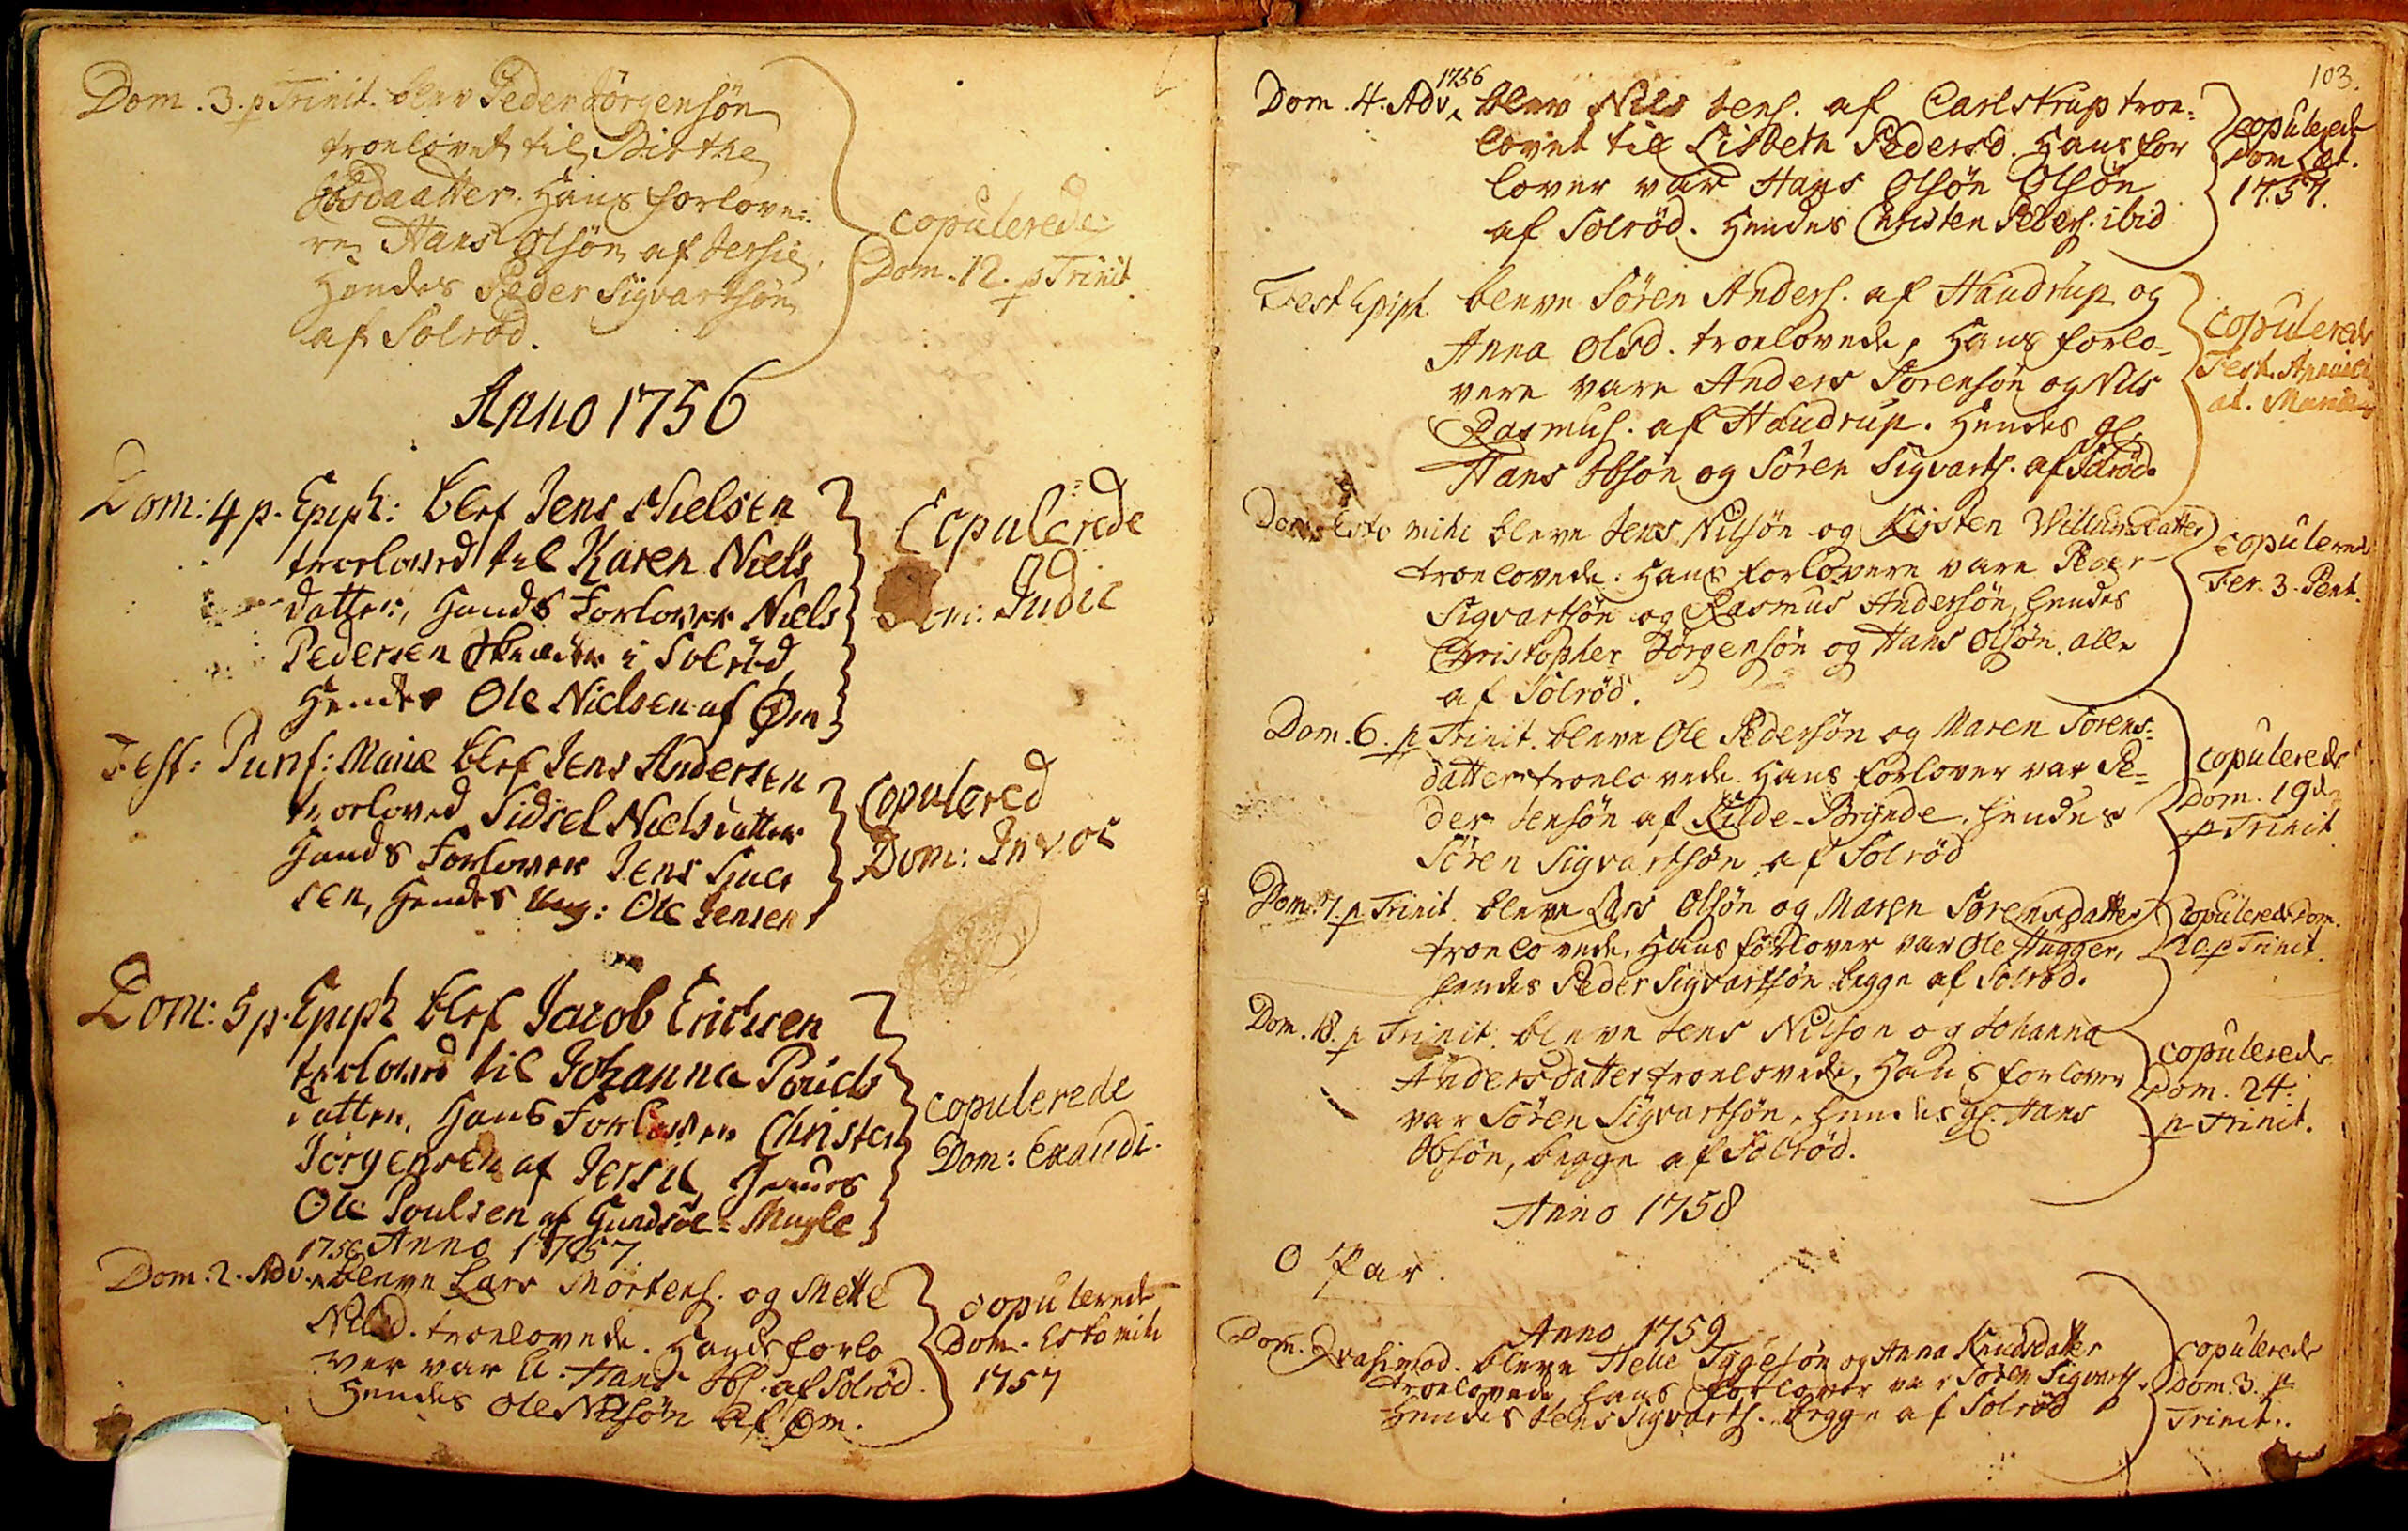Dom. 3. p Trinit. blev Peder Jørgensøn
troelovet til Birthe
Ibsdaatter. Hans Forlove¬
re Hans Olsøn, af Jersie,
Hendes Peder Sigvarsøn
af Solrød.
copulerede
Dom. 12. p Trinit
Anno 1756
Dom: 4 p. Epiph: blef Jens Nielsen
troeloved til Karen Niels
datter, Hands Forlover Niels
Pedersen Skræder i Solrød,
Hendes Ole Nielsen af Øm
Copulerede
Dom: Judic
Fest: Purif: Mariæ blef Jens Andersen
troeloved Sidsel Nielsdatter
Hands Forlover Jens ##
sen, Hendes Ung: Ole Jensen
Copulered
Dom: Invoc
Dom: 5 p. Epiph blef Jacob Erichsen
troloved til Johanna Pouls
datter, Hans Forlover Christen
Jørgensen af Jersie, Hendes
Ole Poulsen af Gundsøe=Magle
Copulerede
Dom: Exaudi.
1756 Anno 1757
Dom. 2. Adv. bleve Lars Mortens. og Mette
Nilsd. troelovede. Hands forlo
ver var U. Hans Ibs. af Solrød
Hendes Ole Nilsøn af Øm.
copulerede
Dom: Estomihi
1757
103.
1756
Dom. 4. Adv blev Nils Jens. af Carlstrup troe¬
lovet til Lisbeth Pedersd. Hans for
lover var Hans Olsøn Olsøn
af Solrød. Hendes Christen Peders. ibid
copulerede
Dom Læt.
1757
Fest Epiph. bleve Søren Anders. af Haudrup og
Anna Olsd. troelovede, Hans forlo¬
vere vare Anders Sørensøn og Nils
Rasmus. af Haudrup. Hendes gl.
Hans Ibsøn og Søren Sigvarts. af Solrød.
Copulerede
Fest. Annunci
at. Mariæ.
Dom. Esto mihi bleve Jens Nilsøn og Kirsten Willumsdatter
troelovede. Hans forlovere vare Peder
Sigvartsøn og Rasmus Andersøn, hendes
Christopher Jørgensøn og Hans Olsøn. Alle
af Solrød.
Copulerede
Fer. 3. Pent.
Dom. 6. p Trinit. bleve Ole Pedersøn og Maren Sørens¬
datter troelovede. Hans forlover var Pe¬
der Jensøn af Kilde-Brynde, hendes
Søren Sigvartsøn af Solrød
Copulerede
Dom. 19de
p Trinit
Dom. 7. p Trinit. bleve Lars Olsøn og Maren Sørensdatter
troelovede. Hans forlover var Ole Hugger,
hendes Peder Sigvartsøn begge af Solrød.
Copulerede Dom.
20. p Trinit.
Dom. 18. p Trinit. bleve Jens Nilsøn og Johanna
Andersdatter troelovede, Hans forlover
var Søren Sigvartsøn, hendes gl. Hans
Ibsøn, begge af Solrød.
Copulerede
Dom. 24.
p Trinit.
Anno 1758
0 Par.
Anno 1759
Dom. Qvasimod. bleve Helle Tygesøn og Anna Knudsdatter
troelovede, hans forlover var Søren Sigvarts.
hendes Jens Sigvarts. begge af Solrød
Copulerede
Dom. 3. p.
Trinit.
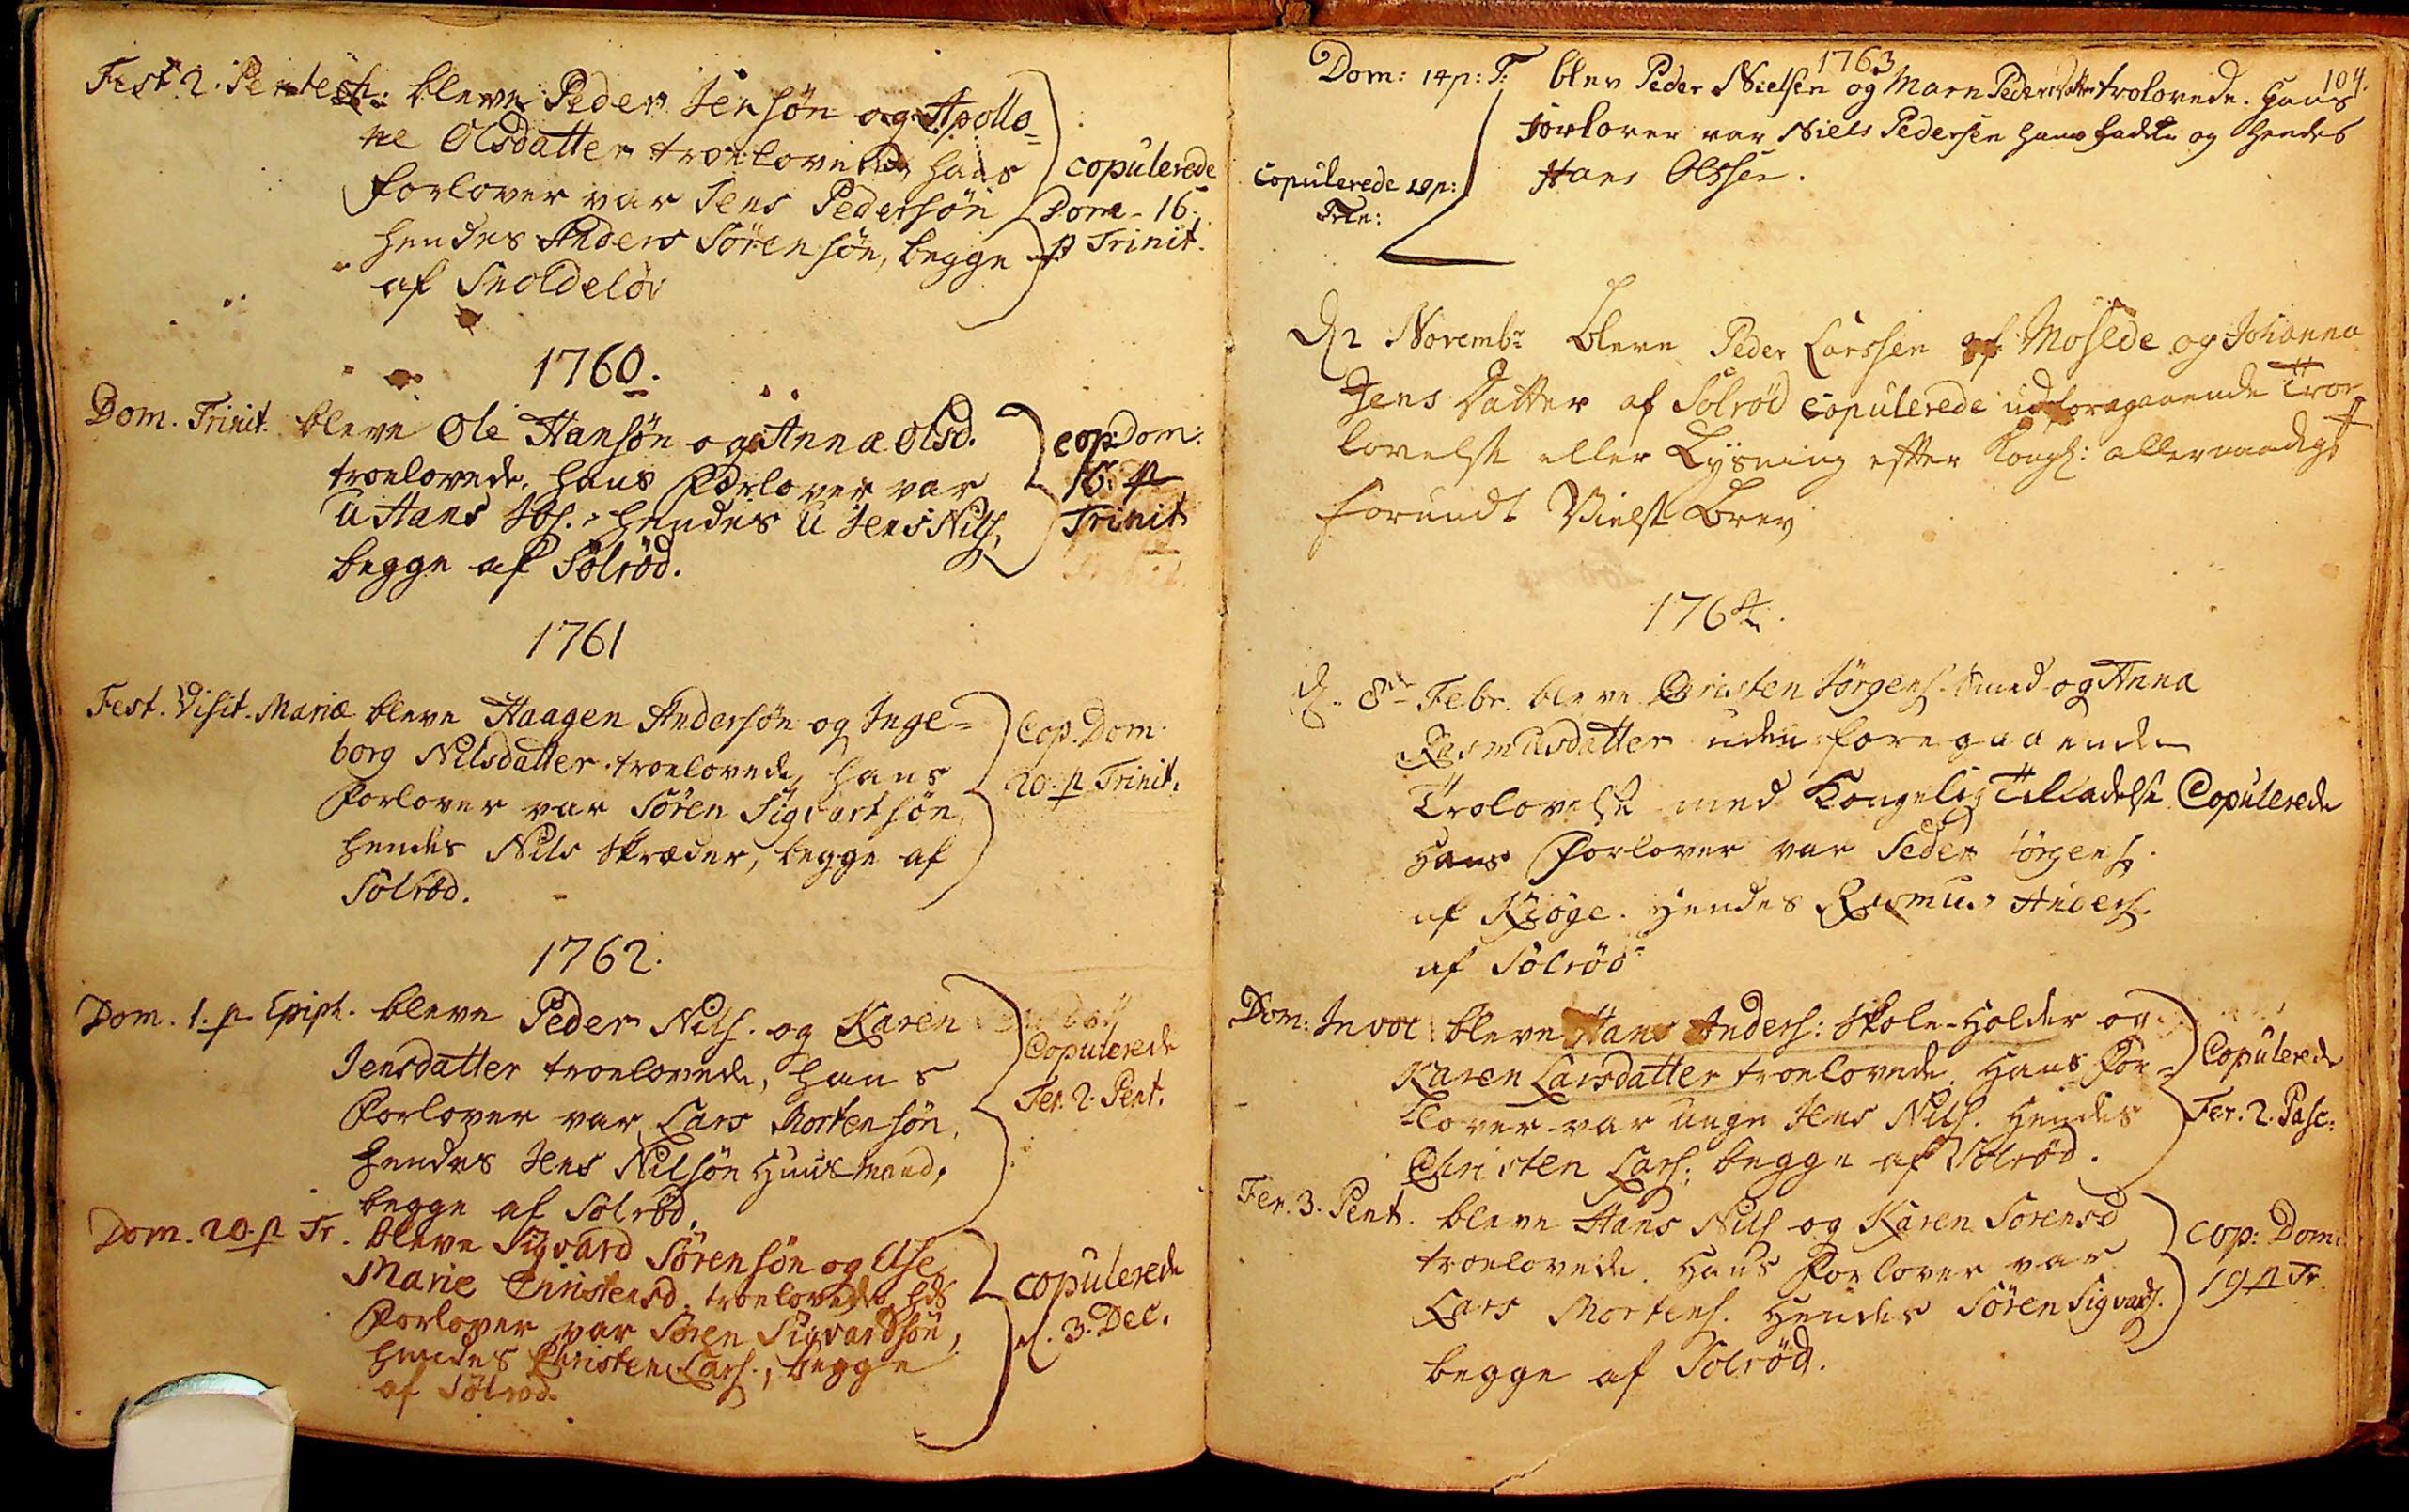Fest. 2. Pentech. bleve Peder Jensøn og Apollo¬
ne Olsdatter troelovede, hans
forlover var Jens Pedersøn
hendes Anders Sørensøn, begge
af Snoldeløv
copulerede
Dom. 16.
p Trinit.
1760.
Dom. Trinit. bleve Ole Hansøn og Anna Olsd.
troelovede, hans Forlover var
U Hans Ibs. hendes U Jens Nils,
begge af Solrød.
cop. Dom:
16: p
Trinit
1761
Fest. Visit. Mariæ bleve Haagen Andersøn og Inge¬           
borg Nilsdatter troelovede, hans
Forlover var Søren Sigvartsøn,
hendes Nils Skræder, begge af
Solrød.
Cop. Dom.
20. p.Trinit.
1762.
Dom. 1. p Epiph. bleve Peder Nils. og Karen
Jensdatter troelovede, hans
Forlover var Lars Mortensøn,
hendes Jens Nilsøn Huusmand,
begge af Solrød.
Copulerede
Fer. 2. Pent.
Dom. 20. p Tr. bleve Sigvard Sørensøn og Else
Marie Christensd. troelovede. hds
Forlover var Søren Sigvardsøn,
hendes Christen Lars., begge
af Solrød.
Copulerede
d. 3. Dec:
104.
1763
Dom: 14 p: T: blev Peder Nielsen og Marn PedersDatter trolovede. Hans
Forlover var Niels Pedersen hans fadder og hendes
Hans Olesen.
Copulerede 28 p:
Trin:
d 2 Novembr bleve Peder Larssen af Mosede og Johanna
Jens Datter af Solrød Copulerede ud foregaaende Troe
lovelse eller Lysning efter Kongl: allernaadigst
forundt Vielse Brev
1764.
d. 8de Febr. bleve Christen Jørgens. Smed og Anna
Rasmusdatter uden foregaaende
Trolovelse med Kongelig Tilladelse Copulerede
Hans Forlover var Peder Jørgens.
af Kiøge. Hendes Rasmus Anders.
af Solrød
Dom: Invoc. bleve Hans Anders: Skole-Holder og
Karen Larsdatter troelovede. Hans For¬  
lover var unge Jens Nils. Hendes
Christen Lars. begge af Solrød.
Copulerede
Fer. 2 Pasc:
Fer. 3. Pent. bleve Hans Nils og Karen Sørensd
troelovede. Hans Forlover var
Lars Mortens. Hendes Søren Sigvads.
begge af Solrød.
Cop: Dom:
19 p Tr